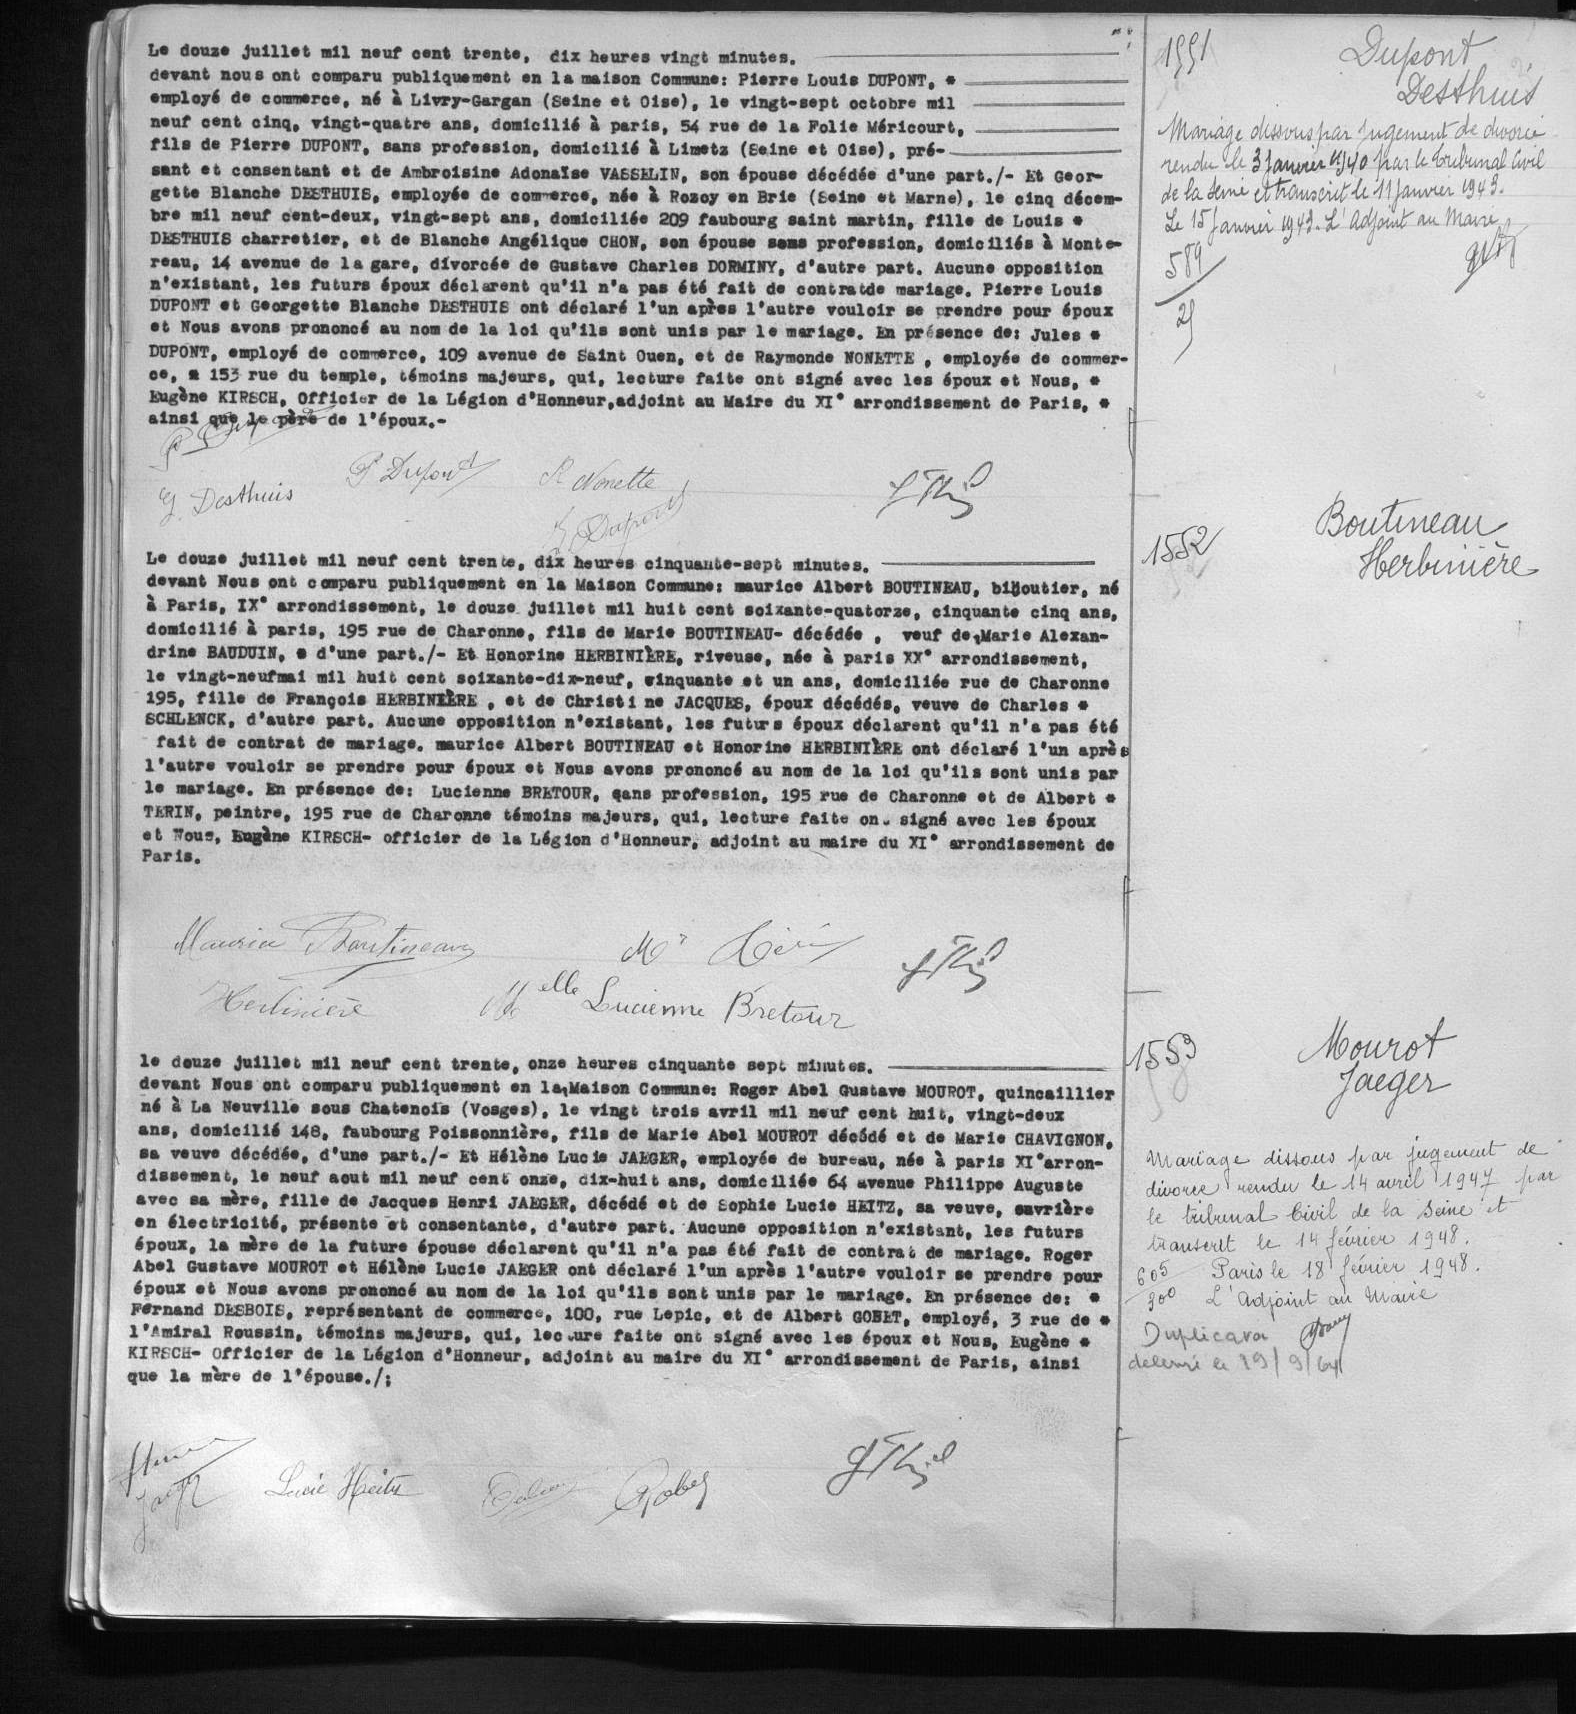Le douze juillet mil neuf cent trente, dix heures vingt minutes.-
devant Nous ont comparu publiquement en la maison Commune: Pierre Louis DUPONT, *-
employé de commerce, né à Livry-Gargan (Seine et Oise), le vingt-sept octobre mil -
neuf cent cinq, vingt-quatre ans, domicilié à paris, 54 rue de la Folie Méricourt, -
fils de Pierre DUPONT, sans profession, domicilié à Limetz (Seine et Oise), pré -
sans et consentant et de Ambroisine Adonaïse VASSELIN, son épouse décédée d'une part ./)-Et Geor-
gette Blanche DESTHUIS, employée de commerce, née à Rozcy en Brie (Seine et Marne), le cinq décem-
bre mil neuf cent-deux, vingt-sept ans, domiciliée 209 faubourg saint martin, fille de Louis *
DESTHUIS charretir, et de Blanche Angélique CHON, son épouse sans profession, domiciliés à Monte-
reau, 14 avenue de la gare, divorcée de Gustave Charle DORMINY, d'autre part. Aucune opposition
n'existant, les futurs époux déclarent qu'il n'a pas été fait de contrat de mariage. Pierre Louis
DUPONT et Georgette Blanche DESTHUIS ont déclaré l'un après l'autre vouloir se prendre pour époux
et Nous avons prononcé au nom de la loi qu'ils sont unis par le mariage. En présence de: Jules *
DUPONT, employé de commerce, 109 avenue de Saint Ouen, et de Raymonde NONETTE, employée de commer-
ce, * 153 rue du temple, témoins majeurs, qui, lecture faite ont signé avec les époux et Nous, *
Eugène KIRSCH, Officier de la Légion d'Honneur, adjoint au Maire du XI ° arrondissement de Paris, *
ainsi que le père de l'époux .-
Le douze juillet mil neuf cent trente, dix heures cinquante-sept minutes.-
devant Nous ont comparu publiquement en la Maison Commune: maurice Albert BOUTINEAU, bijoutier, né
à Paris, IX ° arrondissement, le douze juillet mil huit cent soixante-quatorze, cinquante cinq ans,
domicilié à paris, 195 rue de Charonne, fils de Marie BOUTINEAU-décédée, veuf de Marie Alexan-
drine BAUDUIN, * d'une part ./-Et Honorine HERBINIERE, riveuse, née à paris XX ° arrondissement,
le vingt-neuf mai mil huit cent soixante-dix-neuf, cinquante et un ans, domiciliée rue de Charonne
195, fille de François HERBINIERE, et de Christine JACQUES, époux décédés, veuve de Charles *
SCHLENCK, d'autre part. Aucune opposition n'existant, les futurs époux déclarent qu'il n'a ps été
fait de contrat de mariage. maurice Albert BOUTINEAU et Honorine HERBINIERE ont déclaré l'un après
l'autre vouloir se prendre pour époux et Nous avons prononcé au nom de la loi qu'ils sont unis par
le mariage. En présence de: Lucienne BRETOUR, sans profession, 195 rue de Charonne et de Albert *
TERIN, peintre, 195 rue de Charonne témoins majeurs, qui, lecture faite on signé avec les époux
et Nous, Eugène KIRSCH-officier de la Légion d'Honneur, adjoint au maire du XI ° arrondissement de
Paris.
le douze juillet mil neuf cent trente, onze heures cinquante sept minutes. -
devant Nous ont comparu publiquement en la Maison Commune: Roger Abel Gustave MOUROT, quincaillier
né à La Neuville sous Chatenois (Vosges), le vingt trois avril mil neuf cent huit, vingt-deux
ans, domicilié 148, faubourg Poissonière, fils de Marie Abel MOUROT décédé et de Marie CHAVIGNON,
sa veuve décédée, d'une part ./-Et Hélène Lucie JARGER, employée de bureau, née à paris XI ° arron)
dissement, le neuf aout mil neuf cent onze, dix-huit ans, domiciliée 64 avenue Philipe Auguste
avec sa mère, fille de Jacques Henri JAEGER, décédé et de Sophie Lucie HEITZ, sa veuve, ouvrière
en electricité, présente et consentante, d'autre part. Aucune opposition n'existant, les futurs
époux, la mère de la future épouse déclarent qu'il n'a pas été fait de contrat de mariage. Roger
Abel Gustave MOUROT et Hélène Lucie JAEGER ont déclaré l'un après l'autre vouloir se prendre pour
époux et Nous avons prononcé au nom de la loi qu'ils sont unis par le mariage. En présence de: *
Férnand DESBOIS, représentant de commerce, 100, rue Lepic, et de Albert GOBET, employé, 3 rue de *
l'Amiral Roussin, témoins majeurs, qui, lec ure faite ont signé avec les époux et Nous, Eugène *
KIRSCH-Officier de la Légion d'Honneur, adjoint au maire du XI ° arrondissement de Paris, ainsi
que la mère de l'épouse ./;
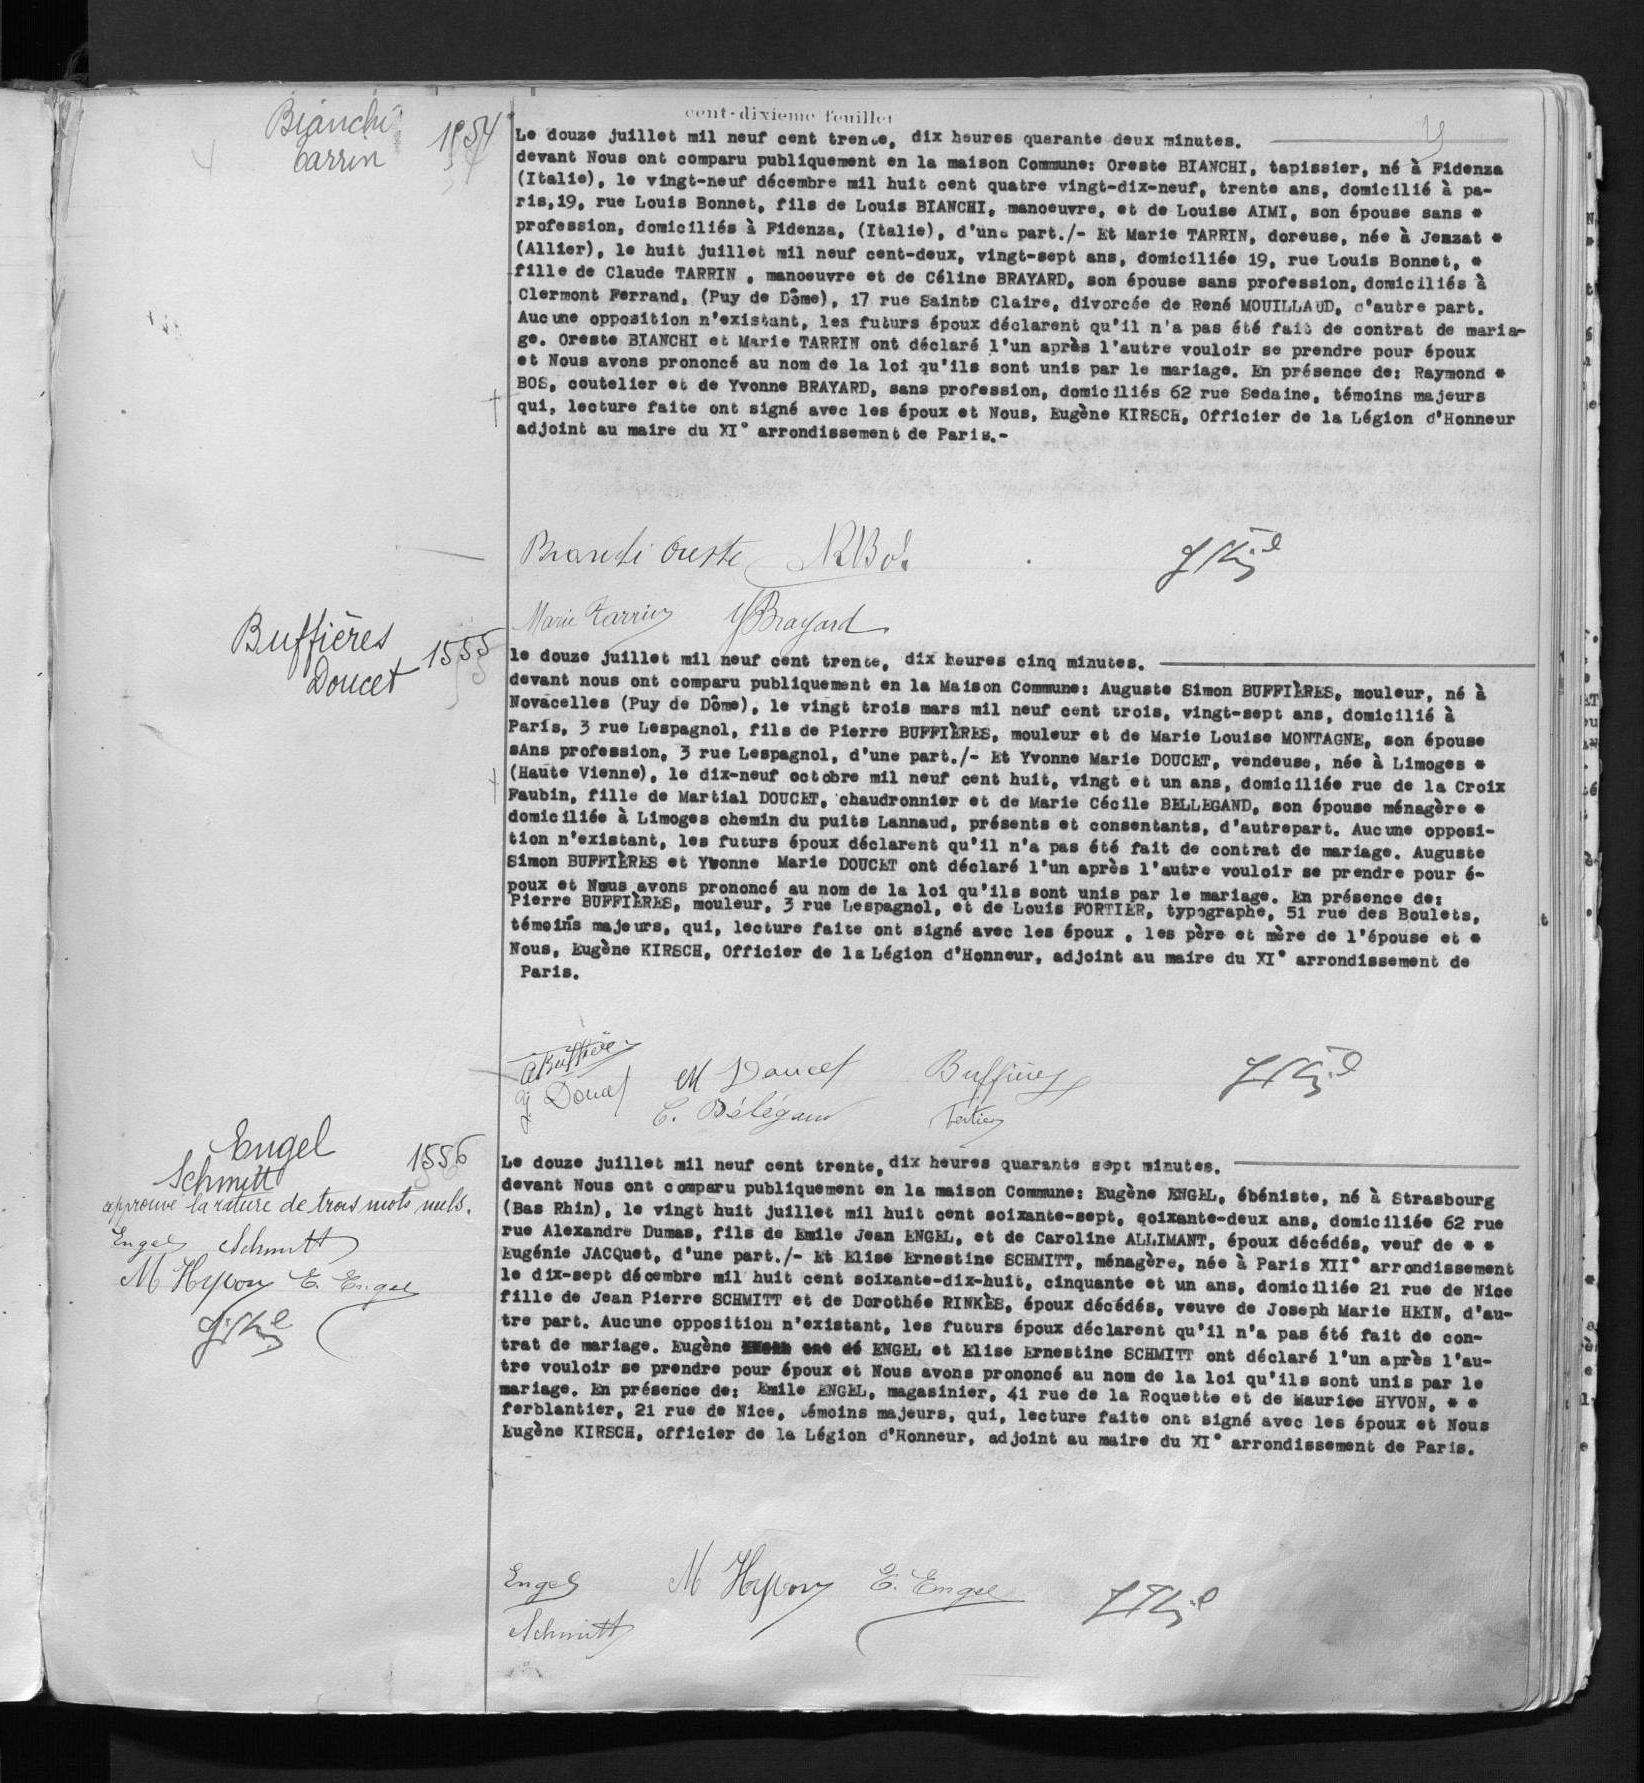Le douze juillet mil neuf cent trente, dix heures quarante deux minutes .-
devant Nous ont comparu publiquement en la maison Commune: Oreste BIANCHI, tapissier, né à Fidenza
(Italie), le vingt-neuf décembre mil huit cent quatre vingt-dix-neuf, trente ans, domicilié à pa-
ris, 19, rue Louis Bonnet, fils de Louis BIANCHI, manoeuvre, et de Louise AIMI, son épouse sans *
profession, domiciliés à Fidenza, (Italie), d'une part ./-Et Marie TARRIN, doreuse, née à Jenzat *
(Allier), le huit juillet mil neuf cent-deux, vingt-sept ans, domiciliée 19, rue Louis Bonnet, *
fille de Claude TARRIN, manoeuvre et de Céline BRAYARD, son épouse sans profession, domiciliés à
Clermont Ferrand, (Puy de Dôme), 17 rue Sainte Claire, divorcée de René MOUILLAUD, d'autre part.
Aucune opposition n'existant, les futurs époux déclarent qu'il n'a pas été fait de contrat de maria-
ge. Oreste BIANCHI et Marie TARRIN ont déclaré l'un après l'autre vouloir se prendre pour époux
et Nous avons prononcé au nom de la loi qu'ils sont unis par le mariage. En présence de: Raymond *
BOS, coutelier et de Yvonne BRAYARD, sans profession, domiciliés 62 rue Sedaine, témoins majeurs
qui, lecture faite ont signé avec les époux et Nous, Eugène KIRSCH, Officier de la Légion d'Honneur
adjoint au maire du XI ° arrondissement de Paris.-
le douze juillet mil neuf cent trente, dix heures cinq minutes .-
devant nous ont comparu publiquement en la Maison Commune: Auguste Simon BUFFIERES, mouleur né à
Novacelles (Puy de Dôme), le vingt trois mars mil neuf cent trois, vingt-sept ans, domicilié à
Paris, 3 rue Lespagnol, fils de Pierre BUFFIERES, mouleur et Marie Louise MONTAGNE, son épouse
sAns profession, 3 rue Lespagnol, d'une part ./-Et Yvonne Marie DOUCET, veudeuse, née à Limoges *
(Haute Vienne), le dix-neuf octobre mil neuf cent huit, vingt et un ans, domiciliée rue de la Croix
Faubin, fille de Martial DOUCET, chaudronnier et de Marie Cécile BELLEGAND, son épouse ménagère *
domiciliée à Limoges chemin du puits Lannaud, présents et consentants, d'autre part. Aucune opposi-
tion n'existant, les futurs époux déclarent qu'il n'a pas été fait de contrat de mariage. Auguste
Simon BUFFIERES et Yvonne Marie DOUCET ont déclaré l'un après l'autre vouloir se prendre pour é-
poux et Nous avons prononcé au nom de la loi qu'ils sont unis par le mariage. En présence de:
Pierre BUFFIERES, mouleur, 3 rue Lespagnol, et de Louis FORTIER, typographe, 51 rue des Boulets,
témoins majeurs, qui, lecture faite ont signé avec les époux, les père et mère de l'épouse et *
Nous, Eugène KIRSCH, Officier de la Légion d'Honneur, adjoint au maire du XI ° arrondissement de
Paris.
Le douze juillet mil neuf cent trente, dix heures quarante sept minutes.-
devant Nous ont comparu publiquement en la maison Commune: Eugène ENGEL, ébéniste, né à Strasbourg
(Bas Rhin), le vingt huit juillet mil huit cent soixante-sept, soixante-deux ans, domiciliée 62 rue
rue Alexandre Dumas, fils de Emile Jean ENGEL, et de Caroline ALLIMANT, époux décédés, veuf de **
Eugénie JACQuet, d'une part ./-Et Elise Ernestine SCHMITT, ménagère, née à Paris XII ° arrondissement
le dix-sept décembre mil huit cent soixante-dix-huit, cinquante et un ans, domiciliée 21 rue de Nice
fille de Jean Pierre SCHMITT et de Dorothée RINKES, époux décédés, veuve de Joseph Marie HEIN, d'au-
tre part. Aucune opposition n'existant, les futurs époux déclarent qu'il n'a pas été fait de con-
trat de mariage. Eugène ENGEL et Elise Ernestine SCHMITT ont déclaré l'un après l'au-
tre vouloir se prendre pour époux et Nous avons prononcé au nom de la loi qu'ils sont unis par le
mariage. En présence de: Emile ENGEL, magasinier, 41 rue de la Roquette et de Maurice HYVON, **
ferblantier, 21 rue de Nice, témoins majeurs, qui, lecture faite ont signé avec les époux et Nous
Eugène KIRSCH, officier de la Légion d'Honneur, adjoint au maire du XI ° arrondissement de Paris.